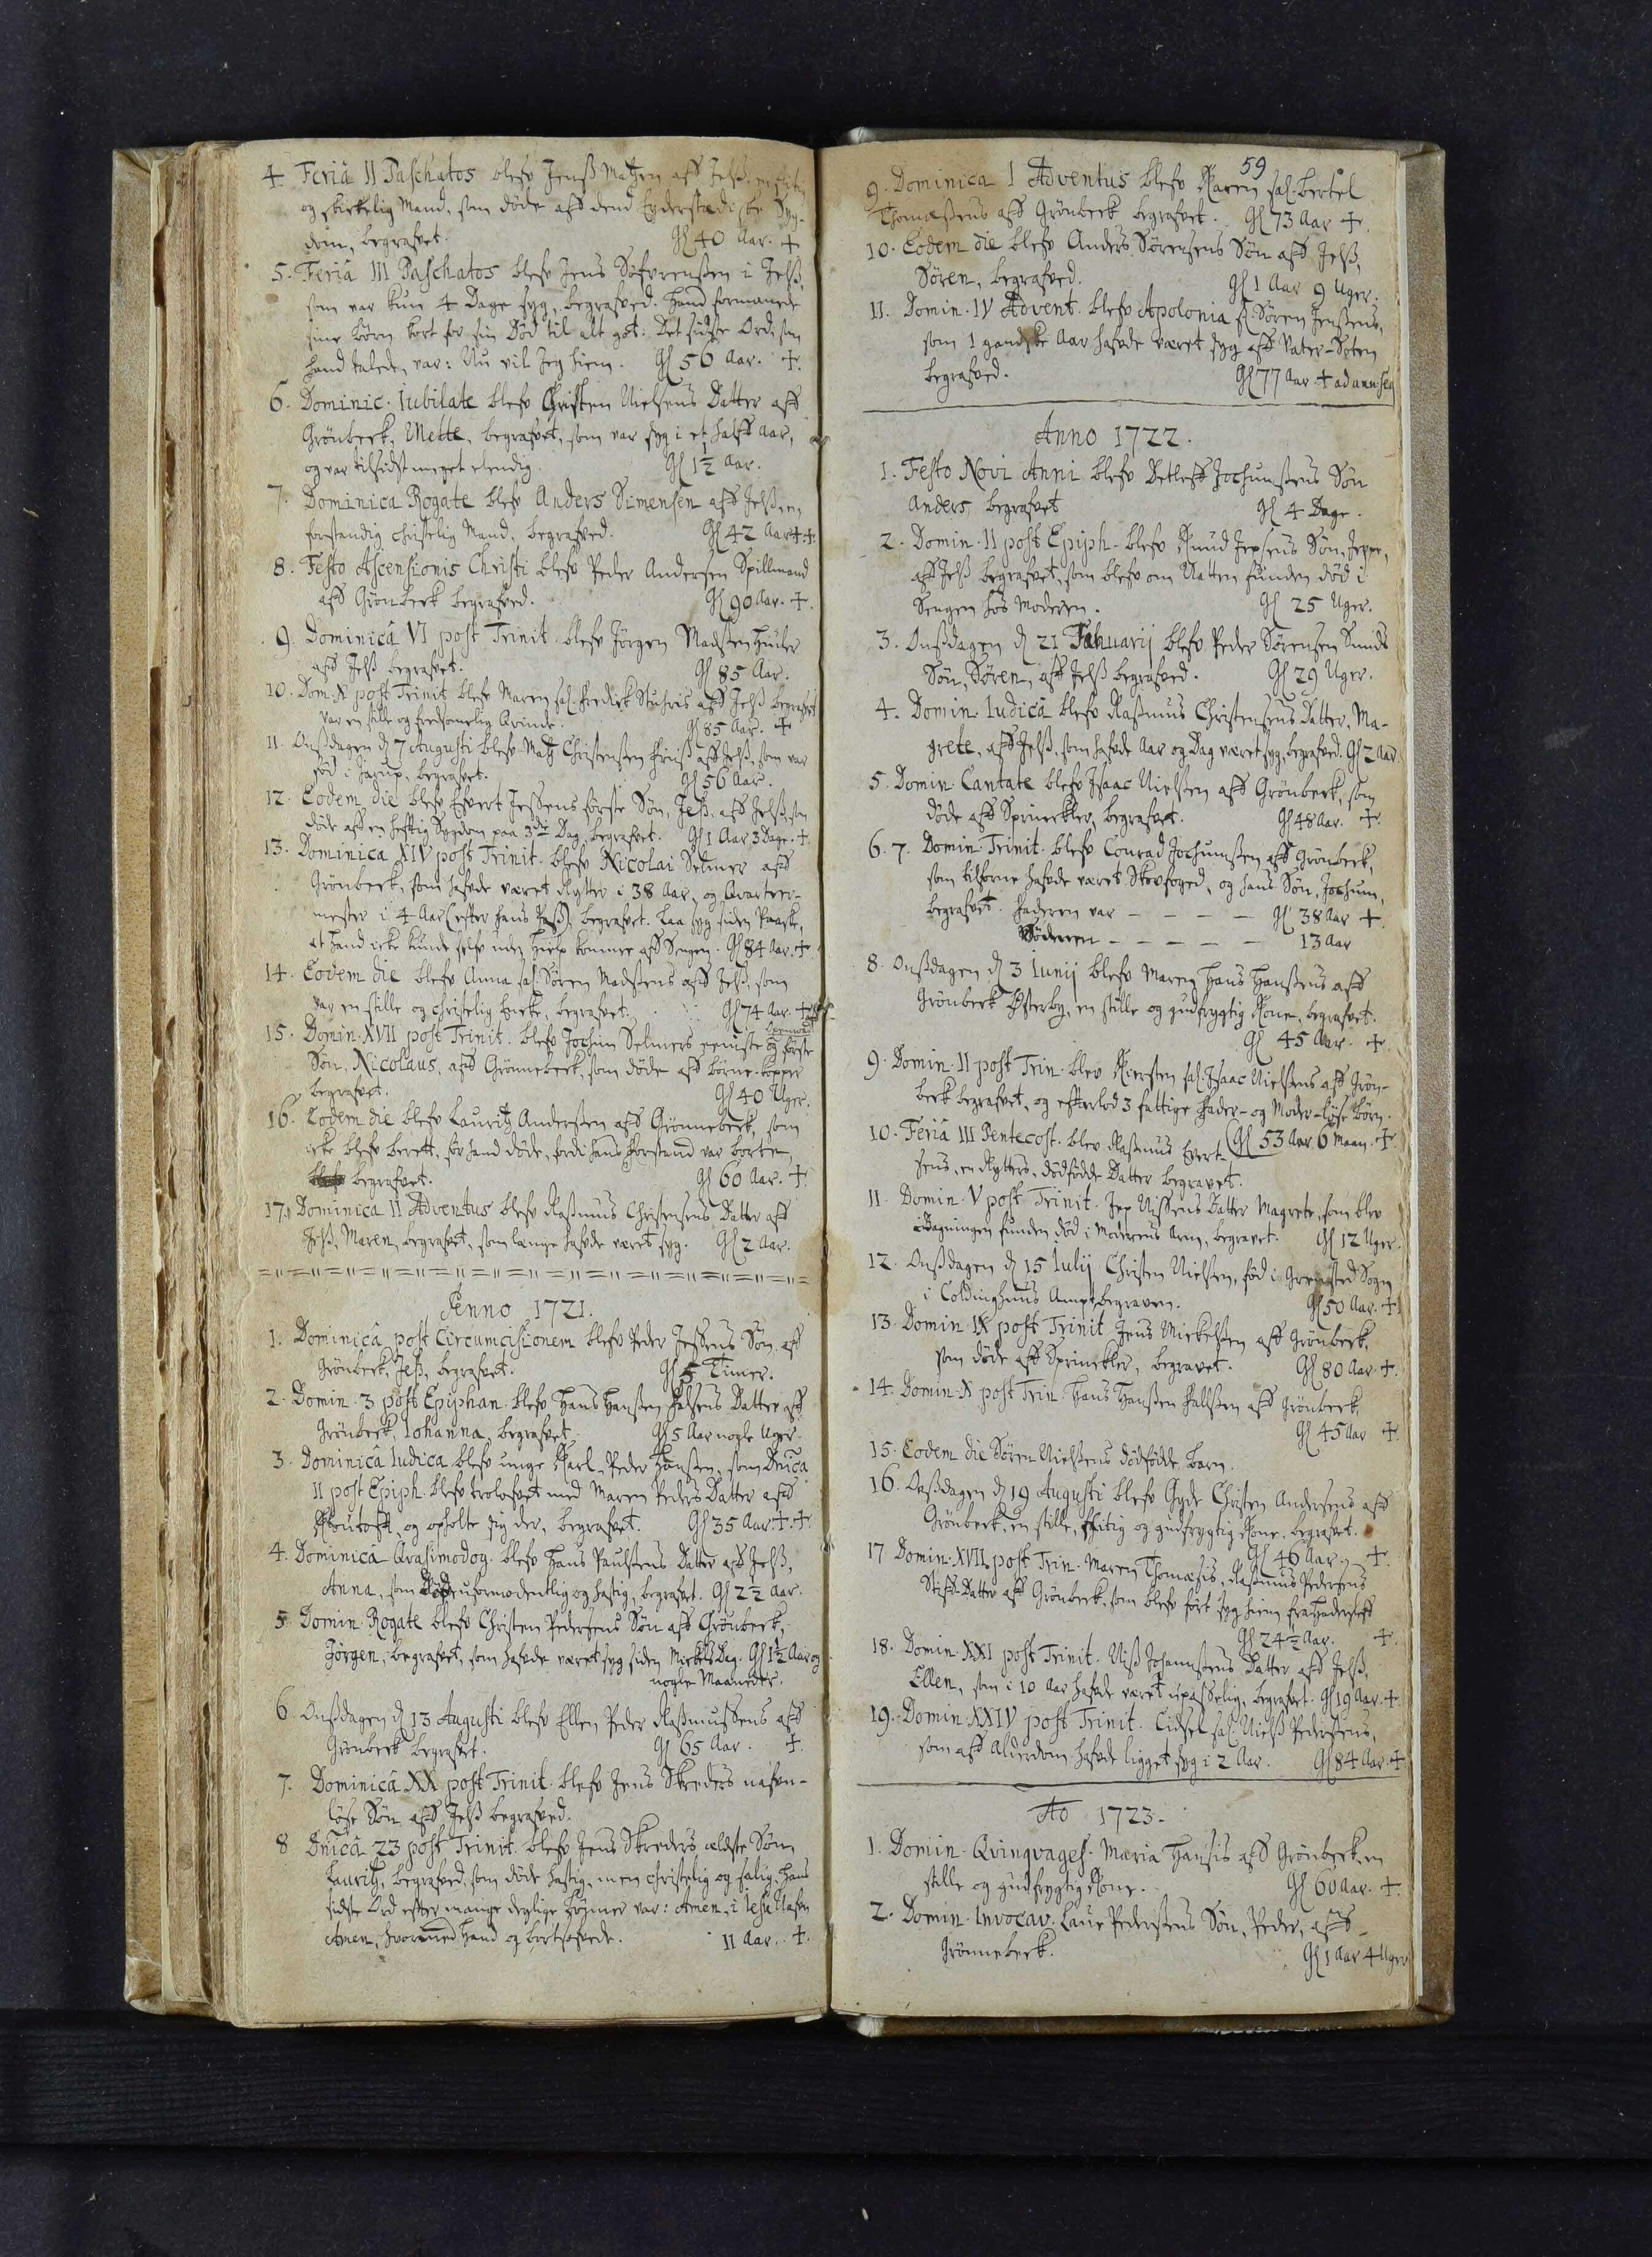4. Feria II Paschatos blefv Jenss Matzen aff Jelss, en flitig
og skickelig Mand, som døde aff dend Eyderstædiske Syg¬
dom, begrafvet.
Gl 40 Aar.
5. Feria III Paschatos blefv Jens Søfrenssen i Jelss,
som var kun 4 Dage syg, begrafved. Hand formanede
sine børn kort for sin Død til alt got. Det sidste Ord, som
hand talede, var: Nu vil Jeg hiem. Gl 56 Aar.
6. Dominic. Iubilate blefv Christen Nielsens Datter aff
Grønbeck, Mette, begrafvet, som var syg i et halvf Aar,
og var tilsidst meget elendig
Gl 1½ Aar.
7. Dominica Rogate blefv Anders Simensen aff Jelss, en
forstandig christelig Mand, begrafved. Gl 42 Aar.
8. Festo Ascensionis Christi blefv Peder Andersen Spillemand
aff Grønbeck begrafved.
Gl 90 Aar.
9. Dominica VI post Trinit. blefv Jørgen Madssen Hiuler
aff Jelss begrafvet.
Gl 85 Aar.
10. Dom. X post Trinit blefv Maren sal. Fredick Stuhris aff Jelss begrafvet
var en stille og fredsommelig Qvinde.
Gl 85 Aar.
11. Onssdagen d 7 Augusti blefv Matz Christenssen Friiss aff Jelss, som var
fød i Jarup, begrafvet.
Gl 56 Aar.
12. Eodem die blefv Efvert Jessens første Søn, Jess, aff Jelss, som
døde aff en hastig Sygdom paa 3die Dag, begrafvet. Gl 1 Aar 3 Dage.
13. Dominica XIV post Trinit. blefv Nicolai Selmer aff
Grønbeck, som hafvde været Rytter i 38 Aar, og Qvartrer¬
mester i 4 Aar (efter hans Pass) begrafvet. Laa syg siden Paaske,
at hand icke kunde selfv uden Hielp komme aff Sengen. Gl 84 Aar.
14. Eodem die blefv Anna sal. Søren Madssens aff Jelss, som
var en stille og christelig Encke, begrafvet.
Gl 74 Aar †
Oxenwad##
15. Domin. XVII post Trinit. blefv Jochim Selmers eeniste og første
Søn, Nicolaus, aff Grønnebeck, som døde aff børne-kopper
begrafvet.
Gl 40 Uger.
16. Eodem die blefv Lauritz Anderssen aff Grønnebeck, som
icke blefs berett, før hand døde, fordi hans forstand var borte,
blefv begrafet.
Gl 60 Aar.
17. Dominica II Adventus blefv Rassmus Christensens Datter aff
Jelss, Maren, begrafvet, som lange hafvde været syg. Gl 2 Aar.
Anno 1721
1. Dominica post Circumcisionem blefv Peder Jessens Søn aff
Grønbeck, Jess, begrafvet.
Gl 5 Timer.
2. Domin. 3 post Epiphan. blefv Hans Hanssen Falsens Datter aff
Grønbeck, Iohanna, begrafvet.
Gl 5 Aar nogle Uger
3. Dominica Iudica blefv unge Karl Peder Hanssen, som Dnica
II post Epiph blefv trolofvet med Maren Peders Datter aff
Kloutofft, og opholte sig der, begrafvet. Gl 35 Aar.
4. Dominica Qvasimodog. blefv Hans Paulssens Datter aff Jelss,
Anna, som døde uformodentlig og hastig, begrafvet. Gl 2½ Aar.
5. Domin. Rogate blefv Christen Pedersens Søn aff Grønbeck,
Jørgen, begrafvet, som hafvde været syg siden Mickels Dag. Gl 1½ Aar og
nogle Maaneder.
6. Onssdagen d 13 Augusti blefv Ellen Peder Rassmussens aff
Grønbeck begrafvet.
Gl 65 Aar.
7. Dominica XX post Trinit. blefv Jens Skreders nafvn¬
løse Søn aff Jelss begrafved.
8. Dnica 23 post Trinit. blefv Jens Skreders aldste Søn,
Lauritz, begrafved, som døde hastig, men christelig og salig, hans
sidste Ord effter mange deylige Bønner var: Amen, i Jesu Nafvn
Amen, hvormed Hand og bortsofvede.
11 Aar.
59
9. Dominica I Adventus blefv Karen sal. Bertel
Thomæssens aff Grønbeck begrafvet. Gl 73 Aar
10. Eodem die blefv Anders Sørensens Søn aff Jelss,
Søren, begrafved.
Gl 1 Aar 9 Uger
11. Domin. IV Advent blefv Apolonia sl. Søren Jenssens,
som I gandske Aar hafvde været syg aff Vater-Soten
begrafved.
Gl 77 Aar † ad ann seq
Anno 1722
1. Festo Novi Anni blefv Detleff Jochumssens Søn
Anders begrafvet
Gl 4 Dage.
2. Domin. II post Epiph. blefv Knud Jepsens Søn, Jeppe,
aff Jelss begrafvet, som blefv om Natten funden død i
Sengen hos Moderen.
Gl 25 Uger.
3. Onssdagen d 21 Januarij blefv Peder Sørensen Smids
Søn, Søren, aff Jelss begrafved. Gl 29 Uger.
4. Domin. Iudica blefv Rassmus Christensens Datter, Ma¬
grete, aff Jelss, som hafvde Aar og Dag været syg, begrafved. Gl 2 Aar
5. Domin. Cantate blefv Isaac Nielssen aff Grønbeck, som
døde aff Sprinckler, begrafvet.
Gl 48 Aar.
6. 7. Domin. Trinit. blefv Conrad Jochumssen aff Grønbeck,
som tilforne hafvde været Skovfoged, og hans Søn, Jochum,
begrafvet. Faderen var - - - - Gl 38 Aar
Sønnen - - - - - 13 Aar
8. Onssdagen, d 3 Iunij blefv Maren Hans Hanssens aff
Grønbeck Østerby, en stille og gudfrygtig Kone, begrafvet.
Gl 45 Aar
9. Domin. II post Trin. blev Kiersten sal. Isaac Nielssens aff Grøn¬
beck begrafvet, og effterlod 3 fattige Fader- og Moder-løse Børn.
Gl 53 Aar 6 Maan.
10. Feria III Pentecost. blev Rassmus Evert¬
sens, en Rytters, dødfødde Datter begravet.
11. Domin. V post Trinit. Jep Nissens Datter Magrete, som blev
i Dagningen funden død i Moderens Arm, begravet. Gl 12 Uger.
12. Onssdagen d 15 Iulij Christen Nielssen, fød i Grensted Sogn
i Coldinghuus Ampt, begraven.
Gl 50 Aar.
13. Domin IX post Trinit Jens Mickelssen aff Grønbeck,
som døde aff Sprinckler, begravet. Gl 80 Aar.
14. Domin X post Trin Hans Hanssen Fallssen aff Grønbeck.
Gl 45 Aar
15. Eodem die Søren Nielssens dødfødde Barn.
16. Onssdagen d 19 Augusti blefv Gyde Christen Andersens aff
Grønbeck, en stille, flitig og gudfrygtig Kone, begrafvet.
Gl 46 Aar
17. Domin. XVII. post Trin. Maren Thomasis, Rasmus Pedersens
Stiff-Datter aff Grønbeck, som blefv ført syg hiem fra Hadersleff
Gl 24½ Aar.
18. Domin. XXI post Trinit. Niss Johannssens Datter aff Jelss,
Ellen, som i 10 Aar hafvde været upasselig, begrafvet. Gl 19 Aar.
19. Domin. XXIV post Trinit. Cidsel sal. Nielss Pedersens,
som aff Alderdom hafvde ligget syg i 2 Aar. Gl 84 Aar.
Ao 1723.
1. Domin. Qvinqvages. Maria Hansis aff Grønbeck, en
stille og gudfrygtig Kone.
Gl 60 Aar.
2. Domin. Invocav. Laue Pederssens Søn, Peder, aff
Grønnebeck.
Gl 1 Aar 4 Uger